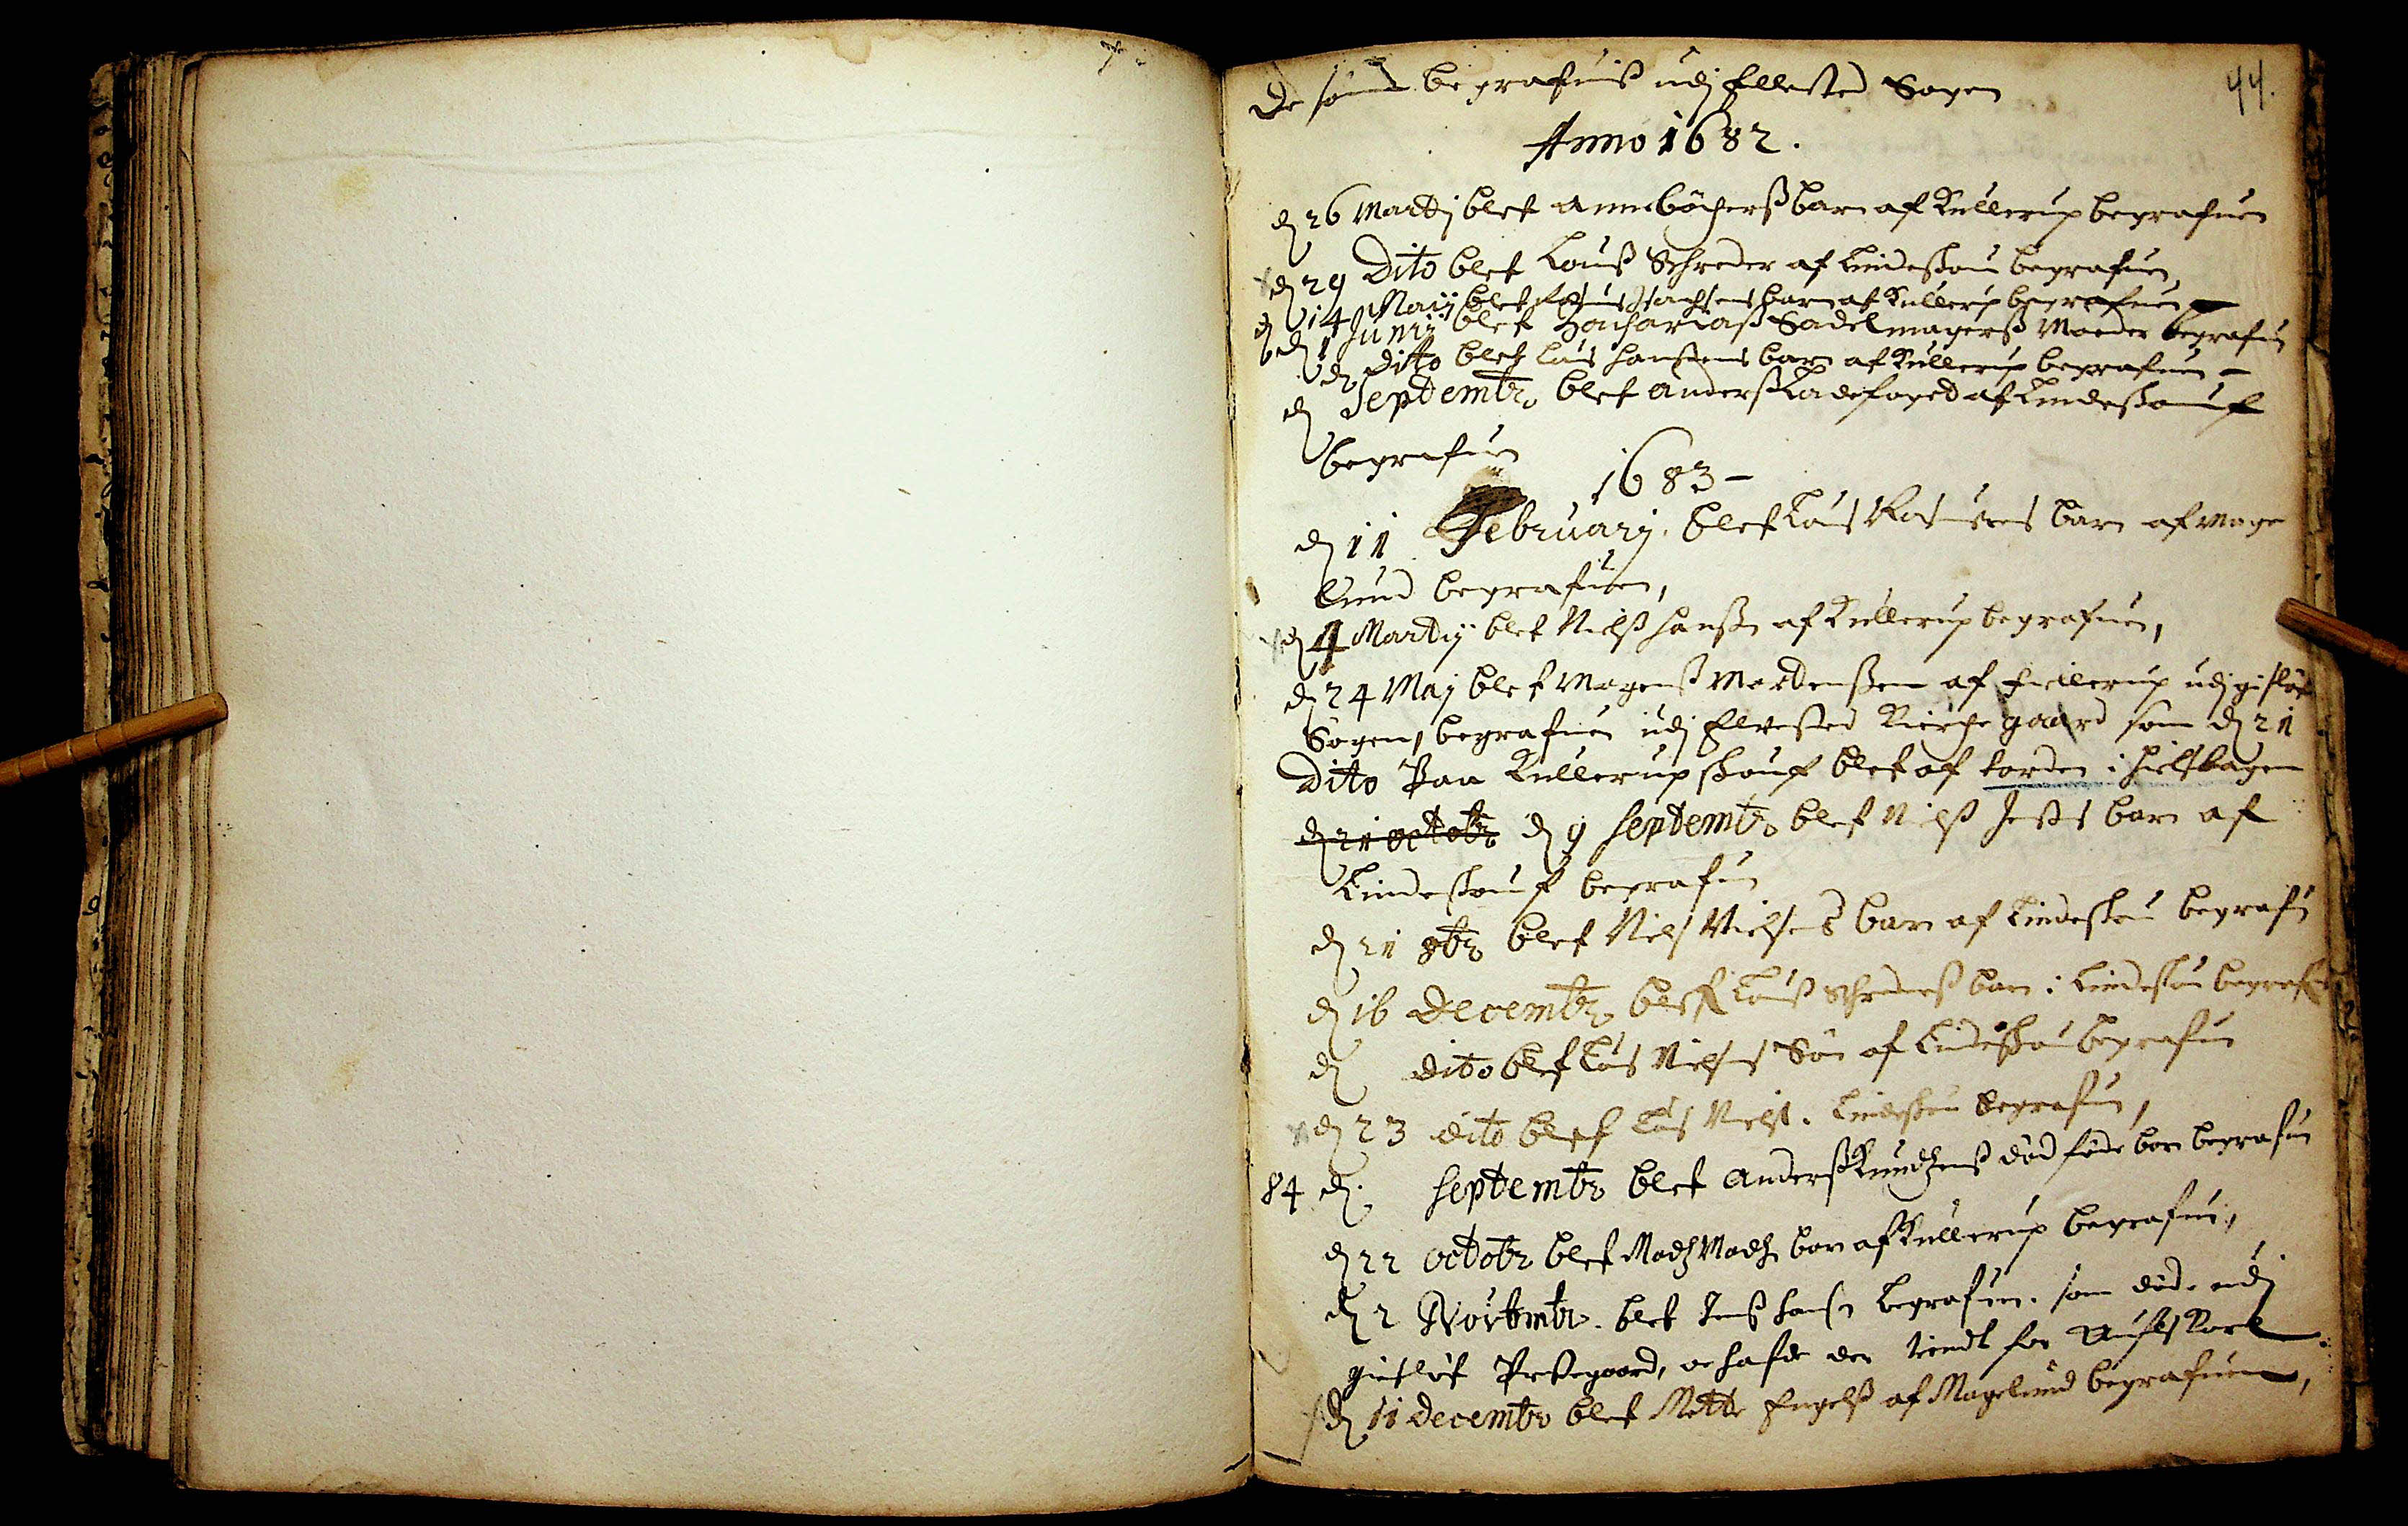44.
De som begrafuis udj Ellested Sogen
Anno 1682.
d 26 Martij blef Anne bøcherss barn af Kullerup begrafuen
d 29 Dito blef Lauss Schreder af Lindeskou begrafuen
d 14 Maij blef  Rasmus Isachsens barn af Kullerp begrafuen
d 1 Junii blef Zachariass Sadelmagerss Moeder begrafuen
d dito blef Laus Hansens barn af Kullerup begrafuen
d Sepdembr. blef Anderss Ladefoget af Lindeskouf
begrafuen
1683
d 11 Februarij. blef Laus Rasmusens barn af Mage
lund begrafuen,
d 4 Martij blef Nielss Hanssen af Kullerup begrafuen
d 24 Maij blef Mogenss Mortenssen af Fiellerup udi gisløf
Sogen begrafuen udi Ellested Kierche gaard som d 21
Dito Paa Kullerup skouf blef af torden i hielbagen
d 21 octobr d 9 Septemb blef Nilss Jenses barn af
Lindeskouf begrafuen
d 21 8br blef Niels Nielses barn af Lindesskou begrafuen
d 16 Decembr blef Lauss Schrederss barn i Lindeskou begraffuen
d dito blef Laus Nielsens Søn af Linderskou begrafuen
d 23 dito blef Laus Niels i Lindeskou begraffuen
84 d. Septembr blef Anderss Knudtzenss død føde barn begrafuen
d 22 octobr blef Mads Mads barn af Kullerup begrafuen,
d 2 Novbmbr. blef Jenss Hansen begrafuen, som døde udj
Gisløf Prestegaard, oc hafde der tiendt for Au##s karl
d 11 Decembr blef Mette Engelss af Magelurd begrafuen
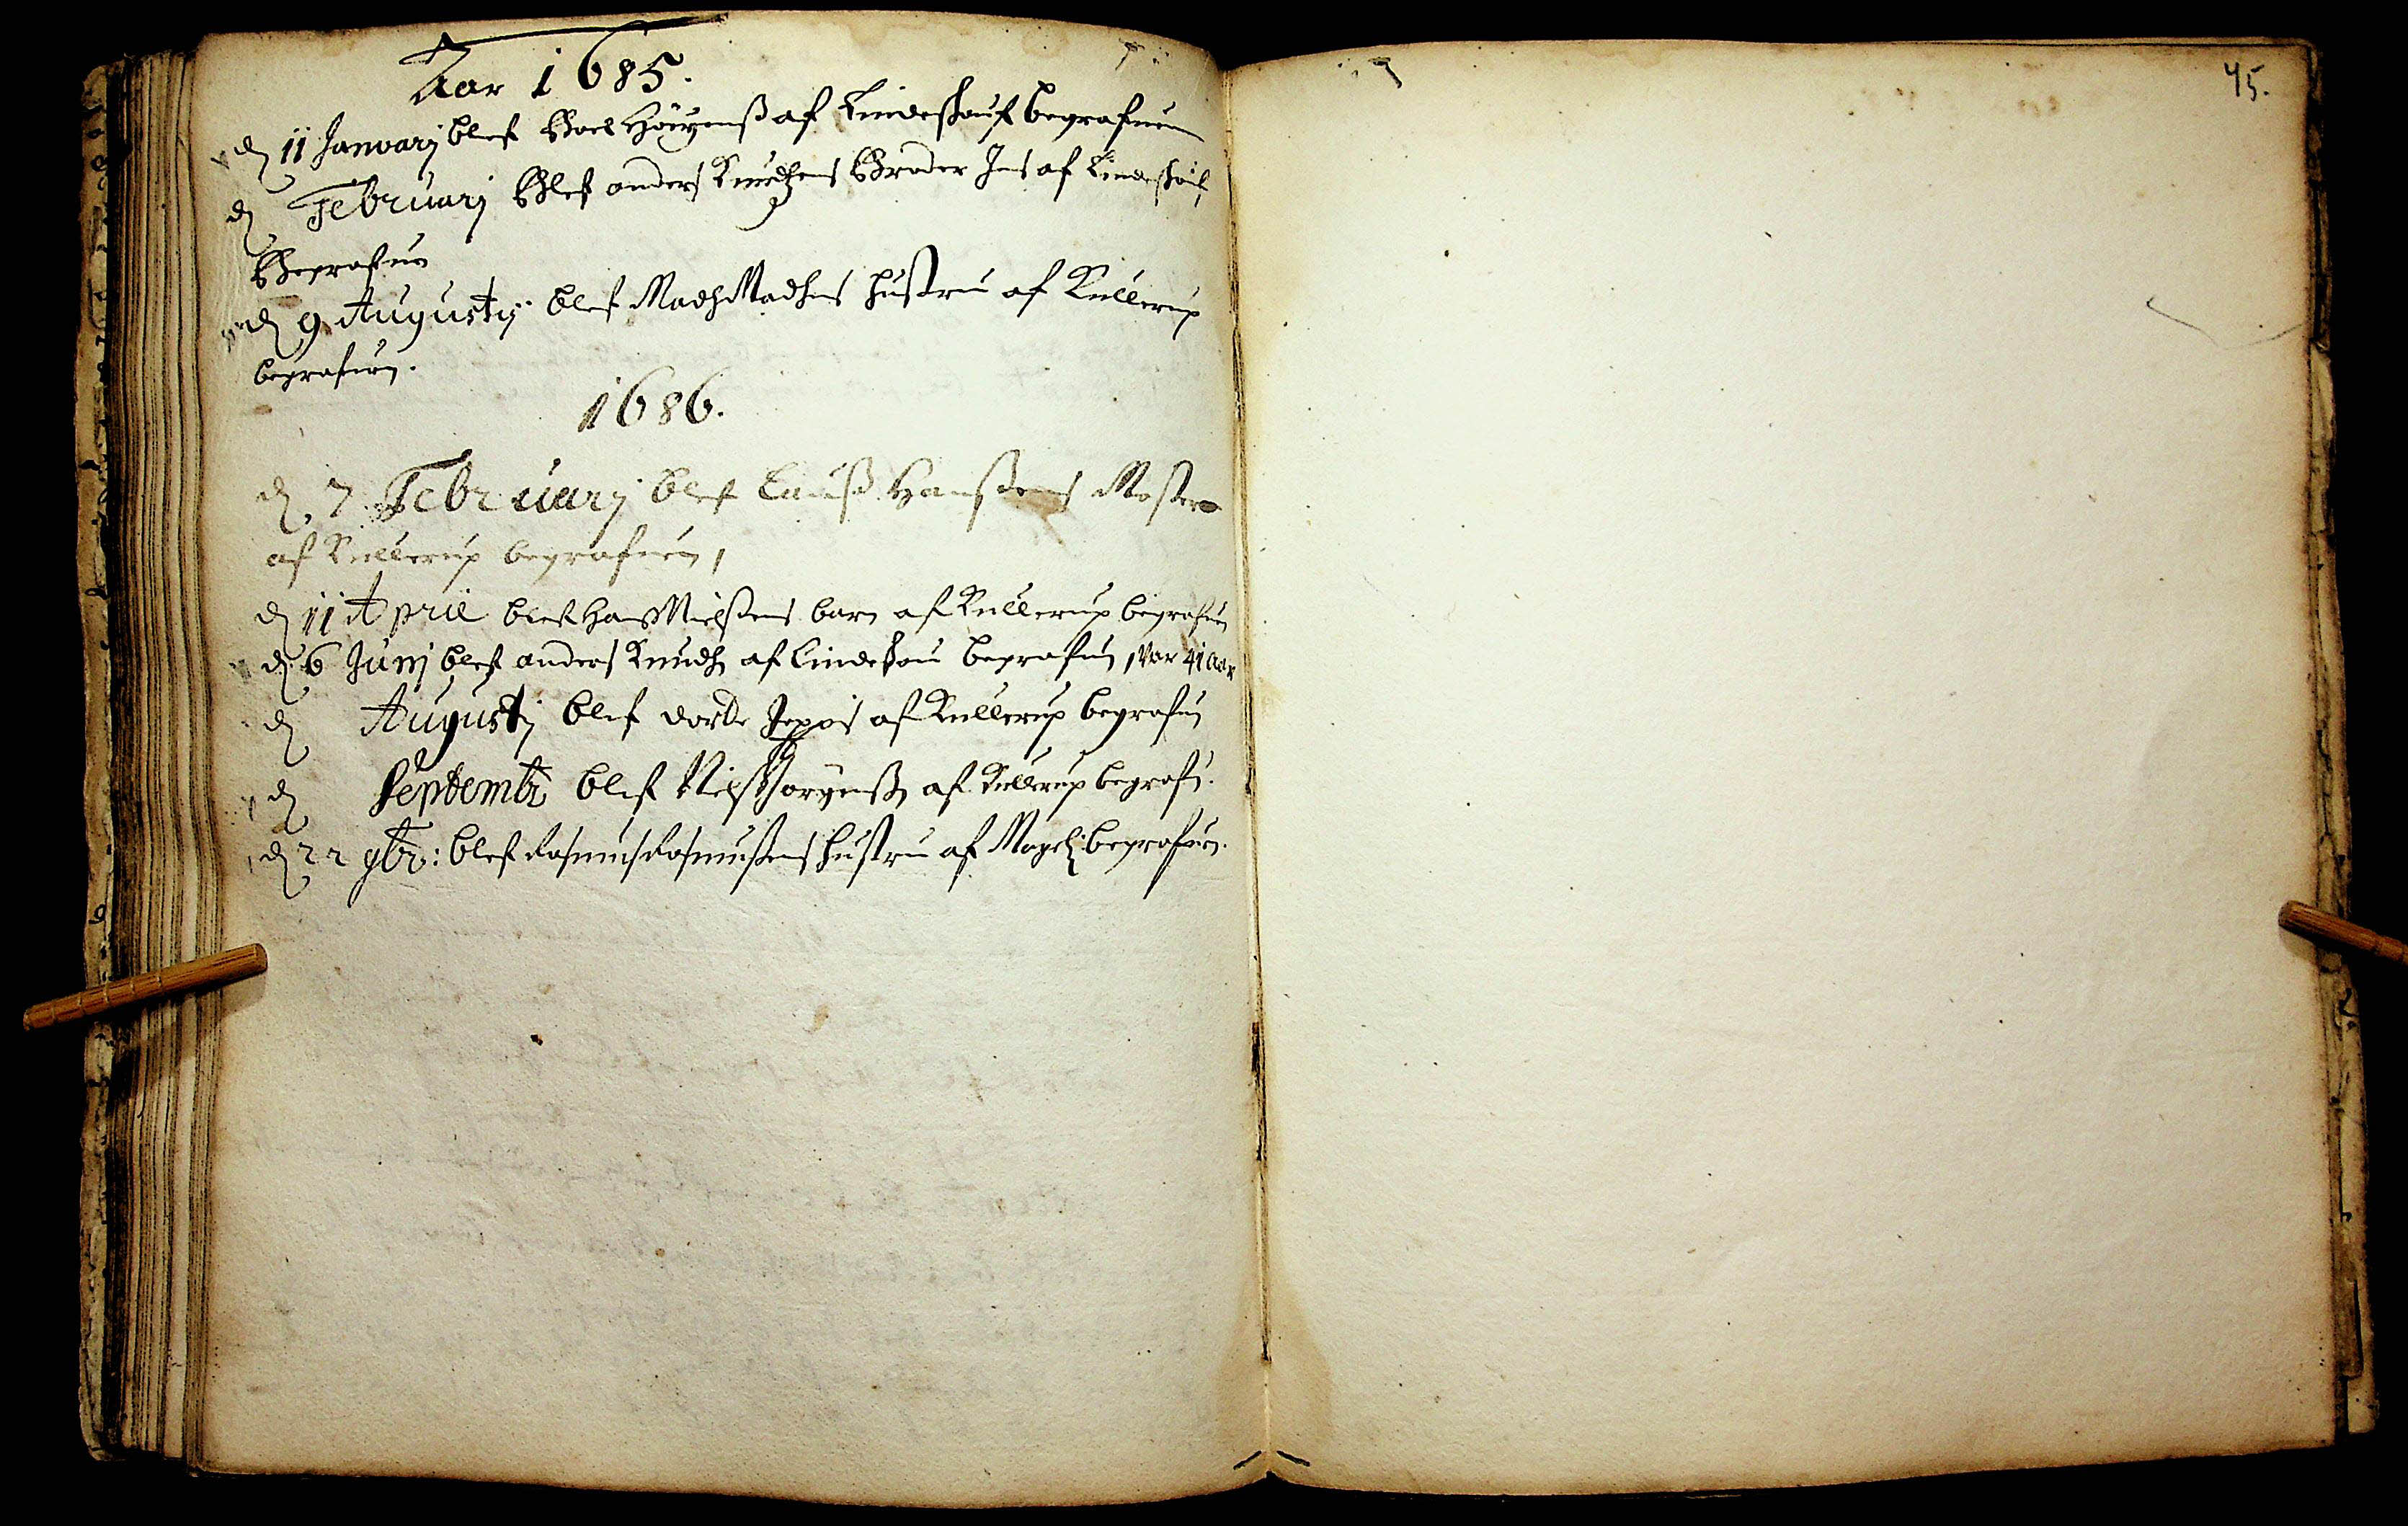Aar 1685.
d 11 Januarij blef Boel Høergenss aff Lindeskouf begraffuen
d Februarij blef Anders Knudtzens Broder Jens aff Lindeskouf
Begrafuen
d 9 Augustij blef Mads Madsens Hustrue aff Kullerup
begrafuen.
1686.
d. 7 Februarij bleff Lauss Hanssens Moster
af Kullerup begrafuen,
d 11 Aprii blef Hans Nielssens barn aff Kullerup begrafuen
d 6 Junij blef Anders Knuds af Lindeskou begraffuen, var 41 Aar
d Augustij blef dorte Jeppis af Kullerup begraffuen
d Septembrs blef Nils Jørgenssen af Kullerup begrafuen
d 22 9br: blef Rasmus Rasmussens Hustru af Magel. begrafuen
45.